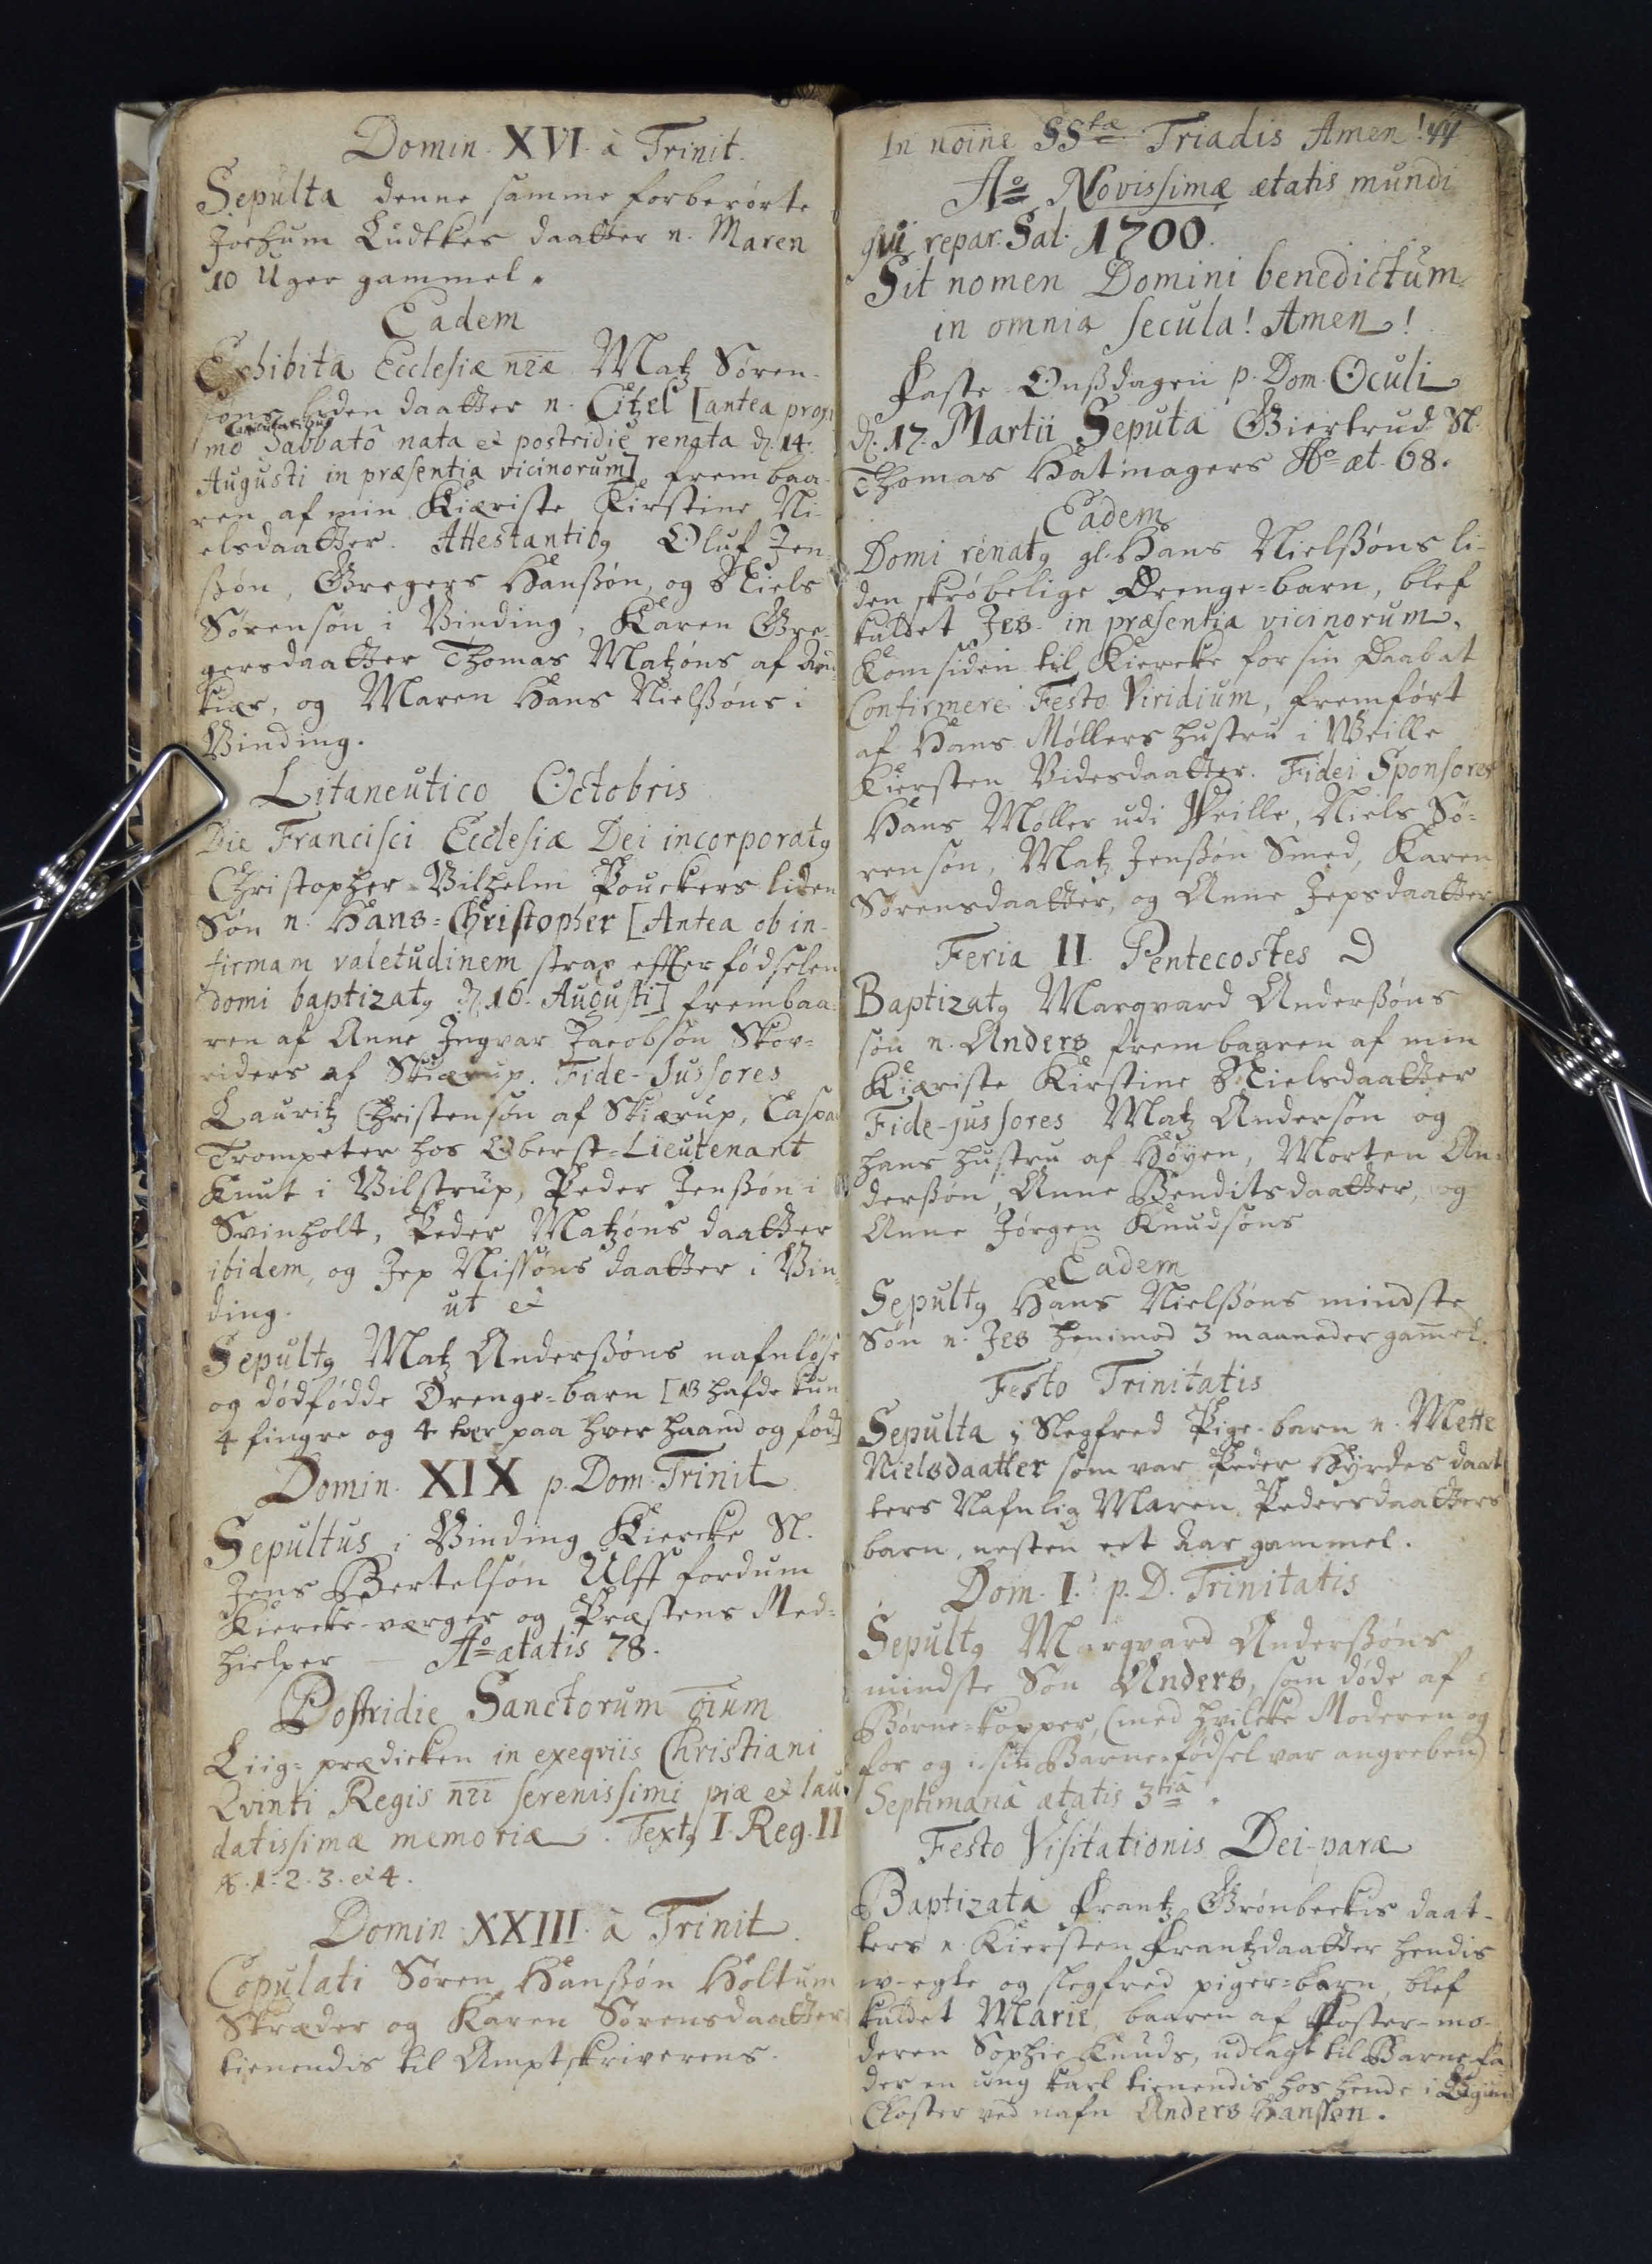Domin. XVI. a Trinit.
Sepulta denne samme forberørte
Jochum Ludtkes daatter n. Maren 
10 uger gammel.
Eadem
Exhibita Ecclesiæ niæ Matz Søren¬
søns liden daatter n. Citzel [antea
mo Sabbato nata et postridie renata d. 14.
Augusti in præsentia vicinorum] frembaa¬
ren af min Kiæriste Kirstine Ni¬
elsdaatter. Attestantibq Oluf Jen¬
søn, Gregers Hanssøn, og Niels
Sørensøn i Vinding, Karen Gre¬
gersdaatter Thomas Matzøns af And
kiær, og Maren Hans Nielssøns i
Vinding.
Litaneutico Octobris
Die Francisci Ecclesiæ Die incorporatq
Christopher Vilhelm Pouckers liden
Søn n. Hans=Christopher [antea ob in
firma m valetudinem strax effter fødselen
domi baptizatq d. 16 Augusti] frembaa¬
ren af Anne Ingvar Jacobsøn Skov¬
riders af Skiærup. Fide-Jussores
Lauritz Christensen af Skiærup, Caspar
Trompeter hos Oberst=Lieutenant
Knut i Vilstrup, Peder Jenssøn i
Svinholt, Peder Matzøns daatter
ibidem, og Jep Nissøns daatter i Vin¬
ding. ut et
Sepultq Matz Anderssøns nafnløse
og dødfødde Drenge=barn [NB. hafde kun
4 fingre og 4 tær paa hver haand og fod]
Domin XIX p. Dom. Trinit
Sepultus i Vinding Kiercke Sl.
Jens Bertelsøn Ulff fordum
Kiercke-værge og Præstens Med¬
hielper
Ao ætatis 78.
Dostridie Sanctorum Tium
Liig=prædicken in exeqviis Christiane
Qvinti Regis nzz## serenidfimi piæ et lau
datissima memoriæ. Texq I Reg. II
1. 2. 3. et 4.
Domin. XXIII. a Trinit
Copulati Søren Hanssøn Holtum
Skræder og Karen Sørensdaatter 
tienendis til Amptskriverens.
44
In noine SStæ Triadis Amen
Ao Novissimæ ætatis mundi
repar. Sat. 1700
Sit nomen Domini benedictum
in omnia secula! Amen!
Faste Onssdagen p. Dom. Oculi
d. 17 Martii Sepulta Giertrud Sl.
Thomas Hatmagers Ao æt. 68.
Eadem
Domi renatq gl. Hans Nielssøns li¬
den skrøbelige Drenge=barn, blef
kaldet Jes in præsentia vicinorum
Kom siden til Kiercke for sin Daab at
Confirmere Festo Viridium, fremført
af Hans Møllers hustru i Weille
Kiersten Videsdaatter. Fidei Sponsores
Hans Møller udi Veille, Niels Sø¬
rensøn, Matz Jenssøn Smed, Karen
Sørensdaatter, og Anne Jepsdaatter.
Feria II Pentecostes d
Baptizatq Marqvard Anderssøns
søn n. Anders frembaaren af min
Kiæriste Kirstine Nielsdaatter
Fide-jussores Matz Andersøn og
hans hustru af Høyen, Morten An¬
derssøn, Anne Benditsdaatter, og
Anne Jørgen Knudsøns
Eodem
Sepultq Hans Nielsøns mindste
Søn n. Jes henimod 3 maaneder gammel.
Festo Trinitatis
Sepulta 1 Slegfred Pige-barn n. Mette
Nielsdaatter som var Peder Hyrdes daat
ters Nafnlig Maren, Pedersdaatters
barn, nesten eet Aar gammel.
Dom. I. p. D. Trinitatis
Sepultq Marqvard Anderssøns
mindste Søn Anders, som døde af
Børne=kopper, (med hvilke Moderen og
for og i sin Barne=fødsel var angreben)
Septimaná ætatis 3tia
Festo Visitationis Dei-paræ
Baptizata Frantz Grønbeckis daat¬
ters n. Kiersten Frantzdaatter hendis
w-egte og slegfred pige=barn, blef
kaldet Marie, baaren af Foster-mo¬
deren Sophie Knuds, udlagt til Barnefa
der en ung karl tienendis hos hende i Lugum
Closter ved nafn Anders Hanssen
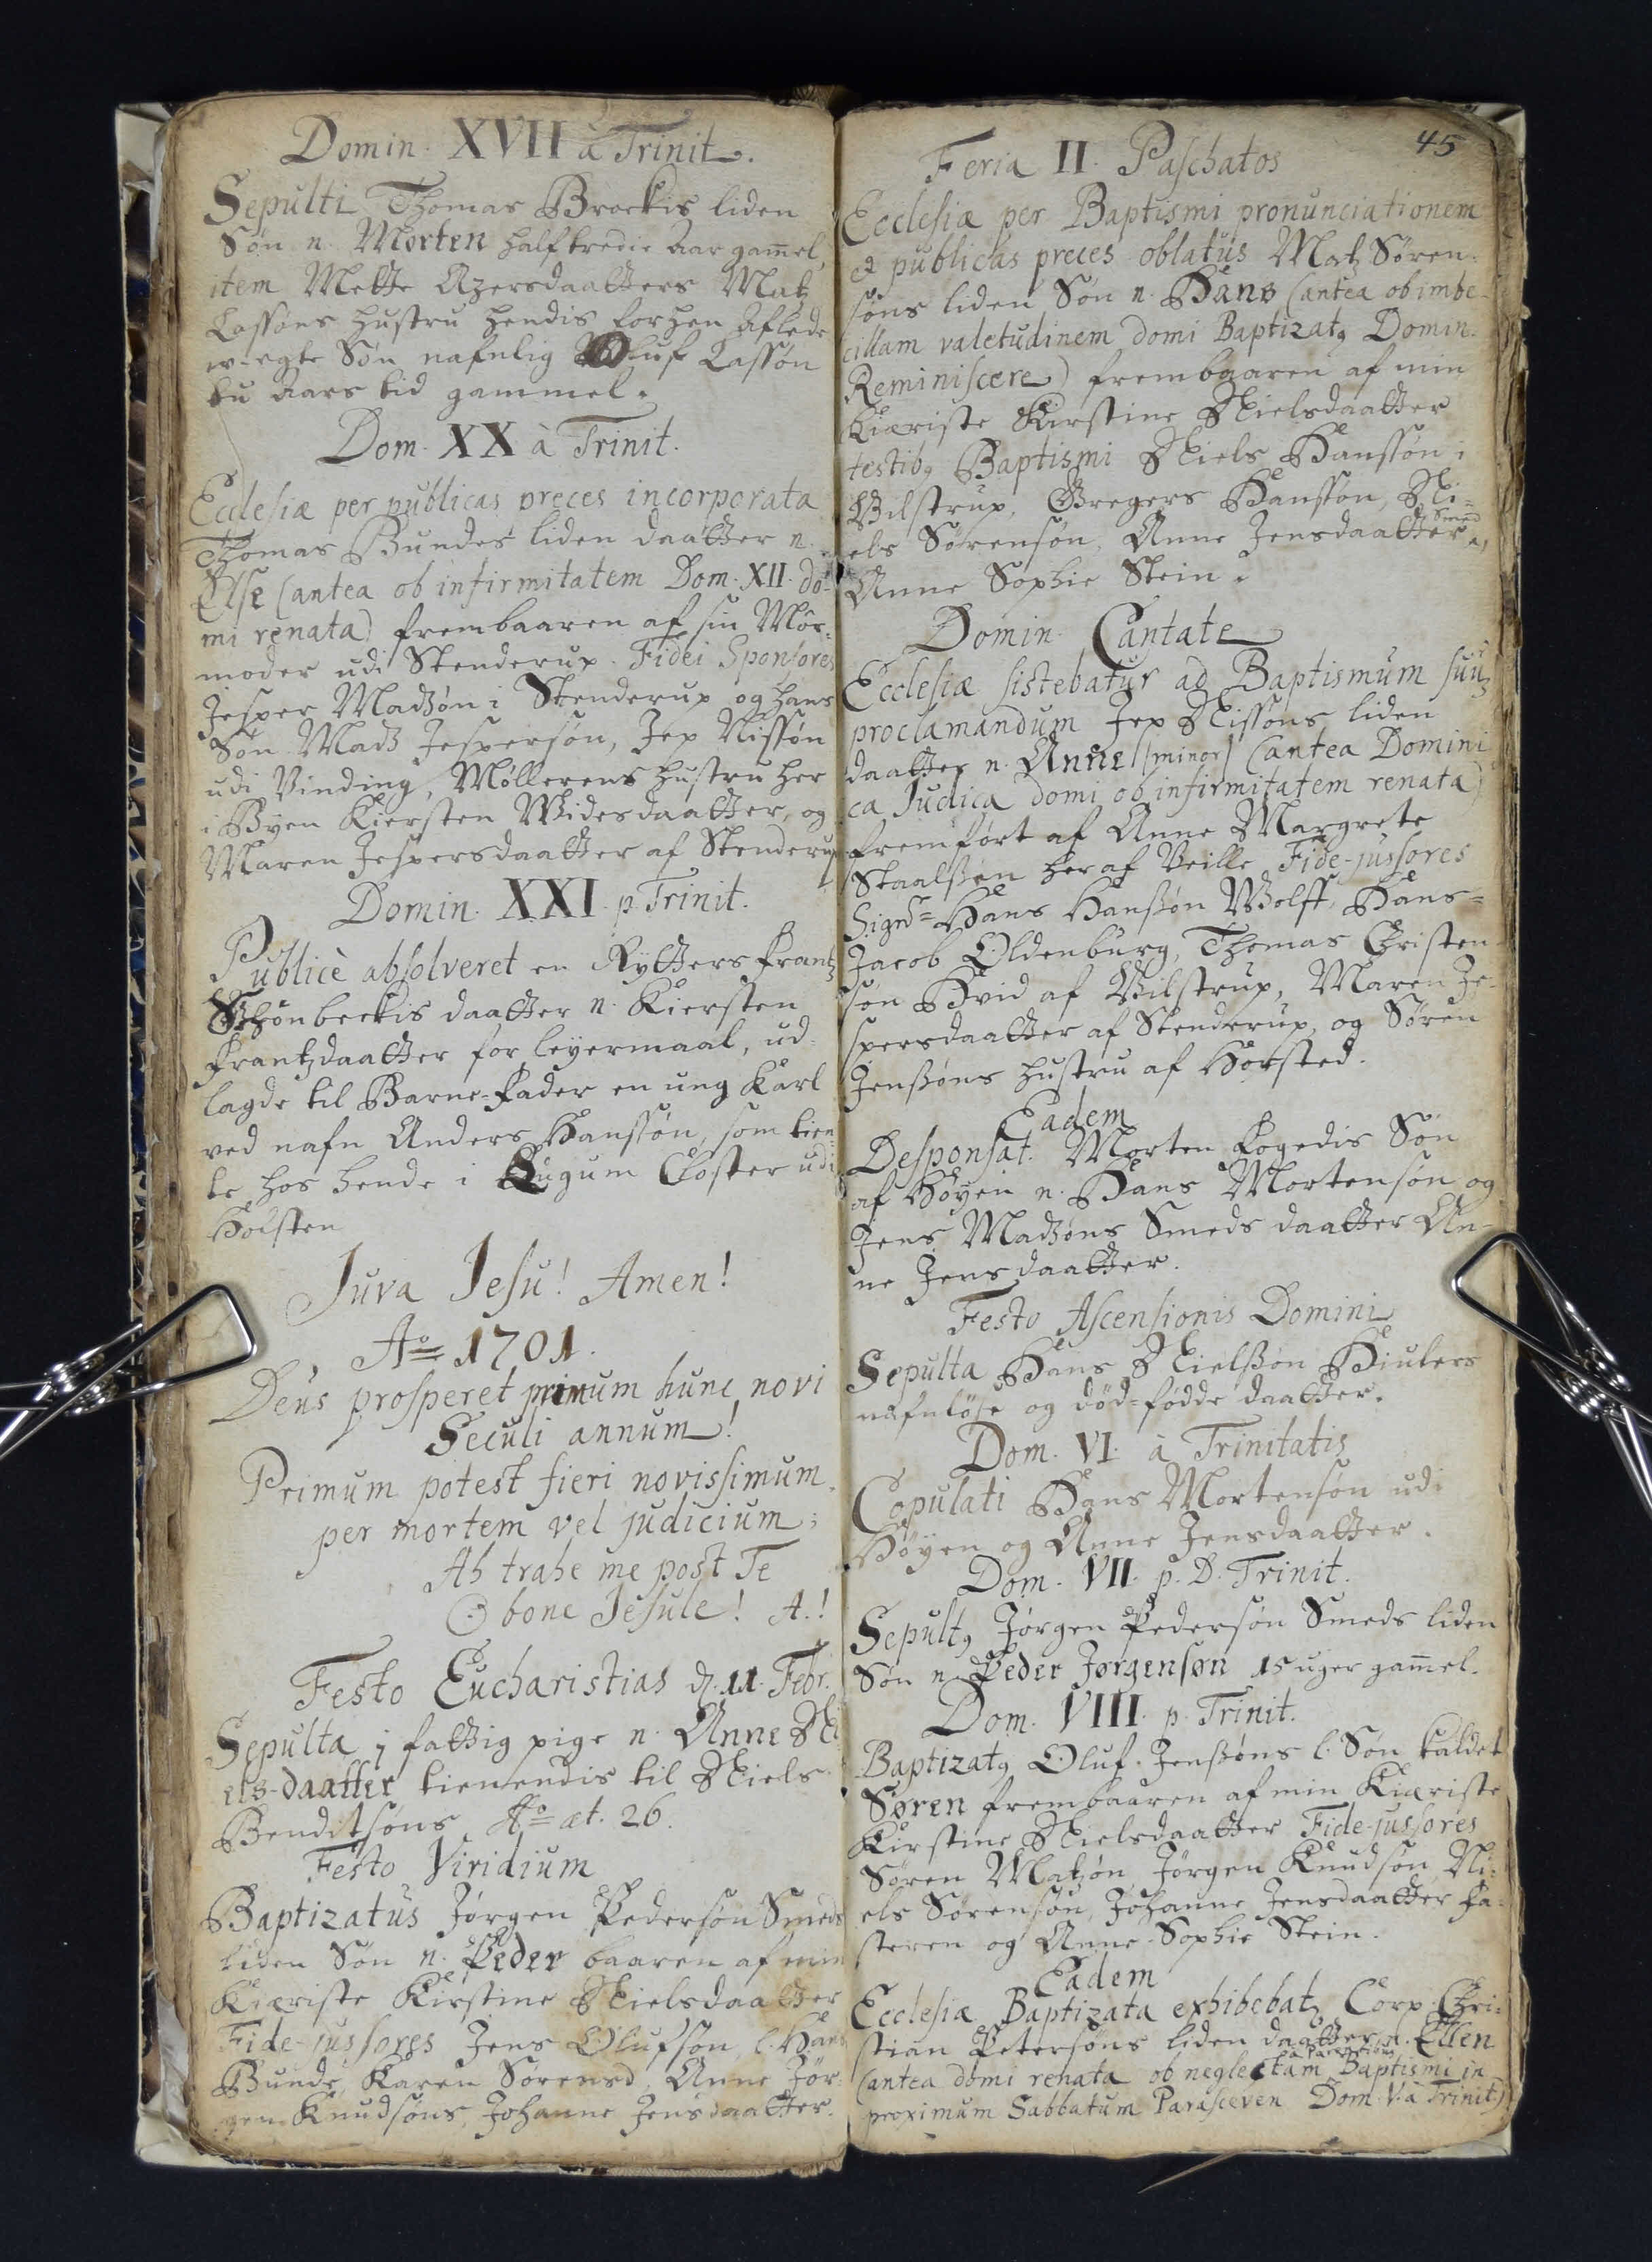Domin. XVII a Trinit
Sepulti Thomas Brockis liden
Søn n. Morten halftredie Aar gamel
item Mette Azersdaatters Matz
Lassøns hustru hendis forhen Aflede
w-egte Søn nafnlig Oluf Lassøn
tu Aars tid gammel.
Dom. XX a Trinit.
Ecclesiæ per publicas preces in corporata
Thomas Bundes liden daatter n. 
Else (antea ob infirmitatem Dom. XII do¬
mi renata) frembaaren af sin Mor¬
moder udi Stenderup. Fidei Sponsores
Jesper Madzøn i Stenderup og hans
Søn Madz Jespersøn, Jep Nissøn
udi Vinding, Møllerens hustru her
i Byen Kiersten Widesdaatter, og
Maren Jespersdaatter af Stenderup
Domin XXI. p. Trinit.
Publicé absolveret en Rytters Frantz
Schønbeckis daatter n. Kiersten
Frantzdaatter for leyermaal, ud¬
lagde til Barne=Fader en ung Karl
ved nafn Anders Hanssøn, som tien¬
te hos hende i Lugum Closter udi
Holsten
Juva Jesu! Aman!
Ao 1701
Deus prosperet primum hune, novi
Seculi annum
Primum potest fieri nivissimum
per mortem vel judicium
Ab trahe me post Te
O bone Jusule! A.!
Festo Eucharistias d. 11 Febr.
Sepulta 1 fattig pige n. Anne Ni¬
els-daatter tienendis til Niels
Benditsøns Ao æt. 26
Festo Viridium
Baptizatus Jørgen Pedersøn Smeds
liden Søn n. Peder baaren af min
Kiæriste Kirstine Nielsdaatter
Fide-jussores. Jens Olufsøn, l. Han 
Bunde Karen Sørensd, Anne Jør
gen Knudsøns, Johanne Jensdaatter.
45
Feria II Paschatos
Ecclesiæ per Baptismi pronunciationem
et publicas preces oblatus Matz Søren¬
søns liden Søn n. Hans (antea obimbe¬
cillam valetudinem domi Baptizatq Domin.
Reminiscere) frembaaren af min
Kiæriste Kirstine Nielsdaatter
Testibq Baptismi Niels Hanssøn i
Vilstrup, Gregers Hanssøn, Ni¬
Smed
els Sørensøn, Anne Jensdaatter.
Anne Sophie Stein.
Domin Cantate
Ecclesiæ sistebatur ad Baptismum suuz
proclamandum Jep Nissøns liden
daatter n. Anne |minor|(antea Domini
ca Judica domi ob infirmitatem renata)
fremført af Anne Margrete
Staalssøn her af Veille Fide-jussores
Signr Hans Hanssøn Wolff, Hans =
Jacob Oldenburg, Thomas Christen
søn Hvid af Vilstrup, Maren Je¬
spersdaatter af Stederup, og Søren
Jenssøns hustru af Horsted.
Eadem
Desponsat. Morten Fogedis Søn
af Høyen n. Hans Mortensøn og 
Jens Madzøns Smeds daatter An¬
ne Jensdaatter
Festo Ascensionis Domini
Sepulta Hans Nielssøn Hiulers
nafnløse og død=fødde daatter.
Dom. VI. a Trinitatis
Copulati Hans Mortensøn udi
Høyen og Anne Jensdaatter
Dom. VII. p. D. Trinit
Sepultq Jørgen Pedersøn Smeds liden
Søn n. Peder Jørgensøn 15 uger gammel.
Dom. VIII. p. Trinit.
Baptizatq Oluf Jenssøns l. Søn kaldet
Søren frembaaren af min Kiæriste
Kirstine Nielsdaatter. Fide-jussores
Søren Matzøn, Jørgen Knudsøn, Ni¬
els Sørensøn, Johanne Jensdaatter Fa¬
steren og Anne - Sophie Stein
Eadem
Ecclesiæ Baptizata exhibebat Corp. Chri¬
stian Petersøns liden daatter n. Ellen
a Parentibus##
(antea domi renata ob neglectam Baptismi in
proximum Sabbatum Parasceven Dom V. a Trinit.)